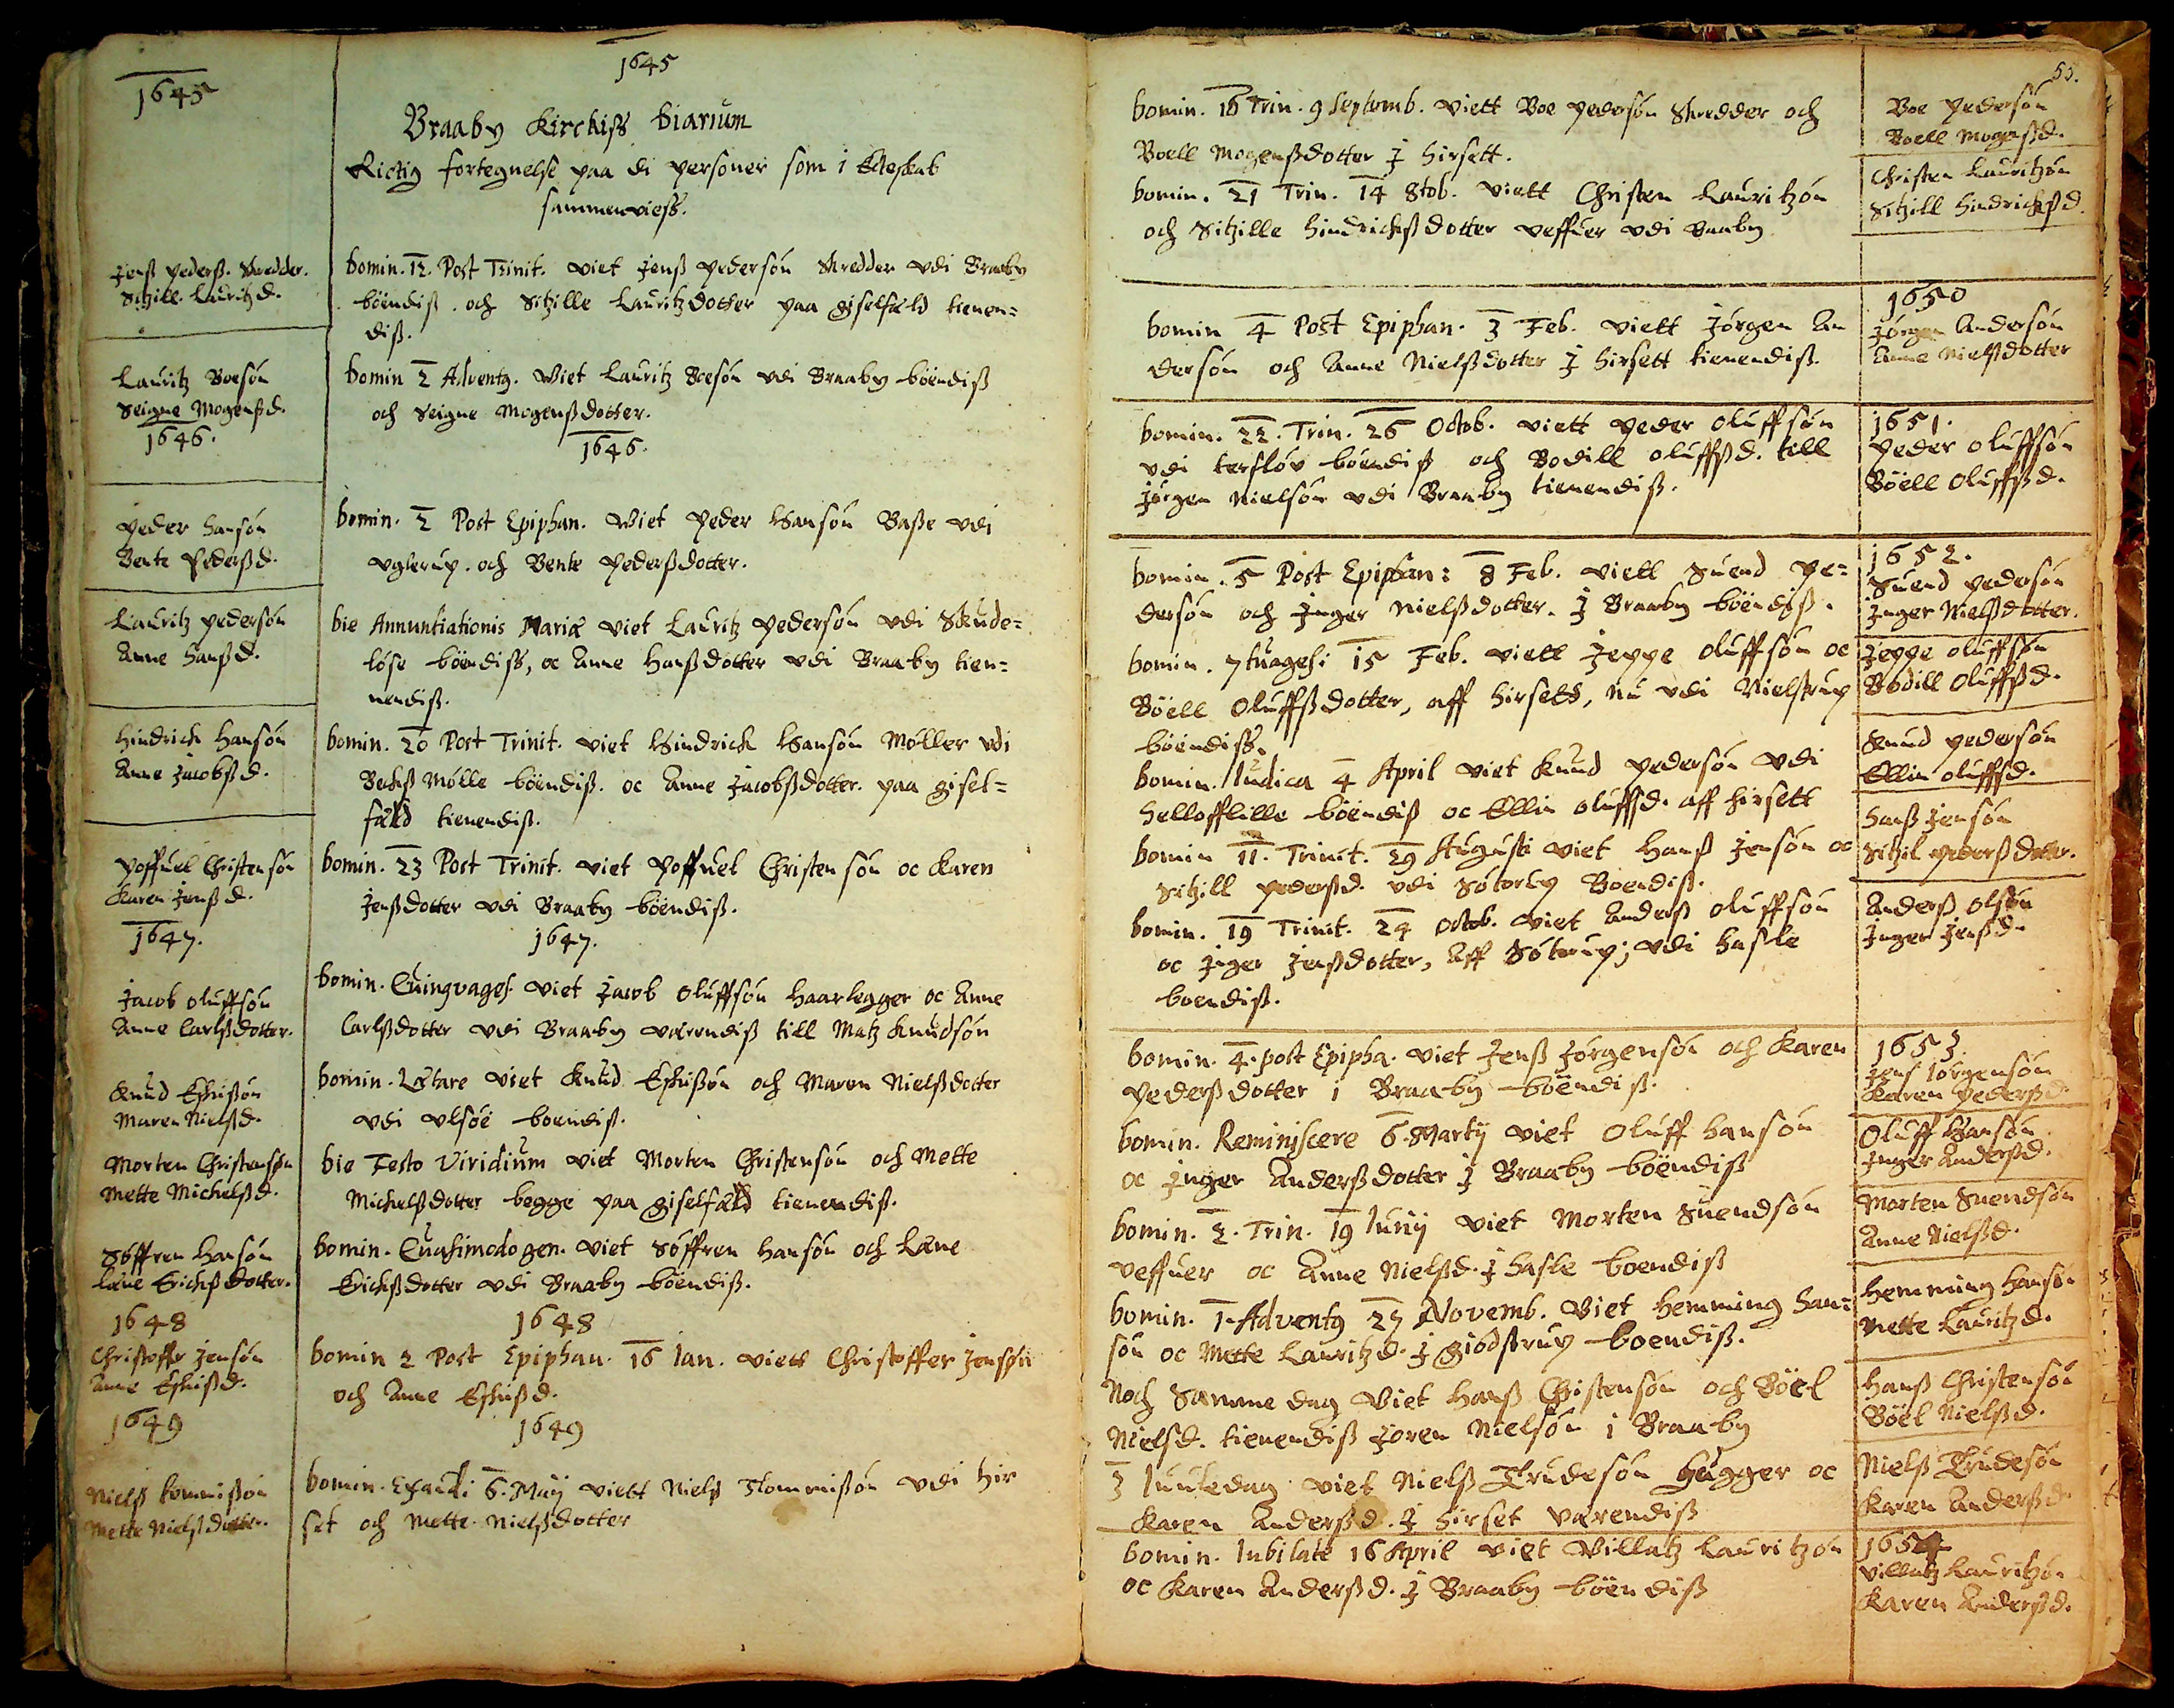1645 
1645 
Braaby Kirchiss Diarium 
Rictig fortegnelse paa di personer som i Ecteskab 
sammenviess.
Jens Peders. Skrædder.
Sitzill. Lauritzd.
Domin. 12. Post Trinit. Viet Jens Pedersøn Skredder Udi Braaby 
boindis och Sitzille Lauritzdotter paa Giselfeld tienen¬
dis. 
Lauritz Boesøn
Seigne Mogensd.
Domin 2 Adventy. Viet Lauritz Boesøn Udi Braaby boendis
och Seigne Mogensdotter. 
1646 
1646 
Peder Hansøn
Bente Pedersd.
Domin. 2 Post Epiphan. Viet Peder Hansøn Basse udi
Uglerup och Bente Pedersdotter.
Lauritz Pedersøn 
Anne Hansd.
Die Annuntiationis Mariæ Viet Lauritz Pedersøn Udi Skude¬
løse boendiss oc Anne Hansdatter Udi Braaby tien¬
nendis.
Hindrich Hansøn 
Anne Jacobsd.
Domin. 20. Post Trinit. Viet Hindrich Hansøn Møller Udi
Becks Mølle boendis. oc Anne Jacobsdotter paa Gisel¬
feld tienendis.
Poffuel Christensøn 
Karen Jensd. 
Domin. 23 Post Trinit. Viet Poffuel Christensøn oc Karen 
Jensdatter Udi Braaby boendis. 
1647.
1647.
Jacob Oluffsøn 
Anne Carlsdatter
Domin. Quinuages. Viet Jacob Oluffsøn Haarlegger## oc Anne
Carlsdotter Udi Braaby værendis till Matz Knudsøn.
Knud Eskisøn
Maren Nielsd.
Domin. Lætare Viet Knud Eskisøn och Maren Nielsdotter 
Udi Ulsøe boendis.
Morten Christensøn 
Mette Michelsd. 
Die Festo Viridium Viet Morten Christensøn och Mette 
Michelsdotter begge paa Giselfeld tienendis. 
Søffren Hansøn 
Læne Erichsdatter.
Domin. Quasimodo gen. Viet Søffren Hansøn och Læne 
Erichsdotter Udi Braaby boendis.
1648 
1648 
Christoffer Jensøn
Anne Eskisd. 
Domin 2 Post Epiphan. 16. Jan. Viett Christoffer Jensøn 
och  Anne Eskisd.
1649 
1649 
Niels Tommisøn 
Mette Nielsdotter.
Domin Exaudi 6. May Viett Niels Thommisøn Udi Hir
set och Mette Nielsdotter
Domin. 16. Trin. 9 Septemb. Viett Boe Pedersøn Skredder och
Boell Mogensdotter J Hirsett.
55.
Boe Pedersøn 
Boell Mogensd.
Domin. 21 Trin. 14 8tob. Viett Christen Lauritzøn 
och  Sitzille Hindrichsdotter Veffuer Udi Braaby.
Christen Lauritzøn 
Sitzille Hindrichsd.
Domin 4 Post Epiphan. 3 Feb. Viett Jørgen An
dersøn och Anne Nielsdotter J Hirsett tienendis.
1650 
Jørgen Andersøn 
Anne Nielsdotter
Domin. 22. Trin. 26 Octob. Viett Peder Oluffsøn 
Udi Tersløv boendis ## och Bodill Oluffsd till
Jørgen Nielsøn Udi Braaby tienendis.
1651 
Peder Oluffsøn 
Boell Oluffsd.
Domin. 5 Post Epiphan: 8 Feb. Viett Suend Pe¬
dersøn och Inger Nielsdotter J Braaby boendis. 
1652.
Suend Pedersøn
Inger Nielsdatter.
Domin. 7tuages: 15 Feb. Viett Jeppe Oluffsøn oc 
Boell Oluffsdotter, aff Hirsett, nu Udi Nielstrup
boendiss.
Jeppe Oluffsøn 
Bodill Oluffsd.
Domin. Judica 4 April Viet Knud Pedersøn Udi 
Hellofflille boendis oc Ellin Olufsd. aff. Hirsett.
Knud Pedersøn 
Ellin Oluffd.
Domin 11. Trinit. 29 Augusti Viet Hans Jensøn oc 
Sitzill Pedersd. Udi Søtorup Boendis.
Hans Jensøn 
Sitzil Pedersdatter. 
Domin. 19 Trinit. 24 Octb. Viet Anders Oluffsøn 
oc Inger Inger Jensd. Aff Søtorup, Udi Hasle
boendis.
Anders Olsøn 
Inger Jensd.
Domin. 4. Post Epiphan. Viet Jens Jørgensøn och Karen 
Pedersdotter i Braaby boendis.
1653
Jens Jørgensøn 
Karen Pedersd. 
Domin. Reminiscere 6. Marty Viet Oluff Hansøn 
oc Inger Andersdotter J Braaby boendis.
Oluff Hansøn 
Inger Andersd.
Domin. 2. Trin. 19 Juny Viet Morten Suendsøn 
Veffuer oc Anne Nielsd. J Hasle boendis. 
Morten Suendsøn 
Anne Nielsd.
Domin. 1. Adventy 27 Novemb. Viet Hemming Han¬
søn oc Mette Lauritzd. J Giodstrup boendis. 
Hemming Hansøn 
Mette Lauritzd.
Noch samme dag Viet Hans Christensøn och Boel 
Nielsd. tienendis Jøren Nielsøn i Braaby.
Hans Christensøn 
Boel Nielsd. 
3 Juuledag Viet Niels Trudesøn Hugger oc 
Karen Andersd. J Hirset værendis. 
Niels ##Trudesøn
Karen Andersd.
Domin. Jubilate 16 April Viet Villatz Lauritzøn 
oc Karen Andersd. J Braaby boendis.
1654
Villatz Lauritzøn 
Karen Andersd.
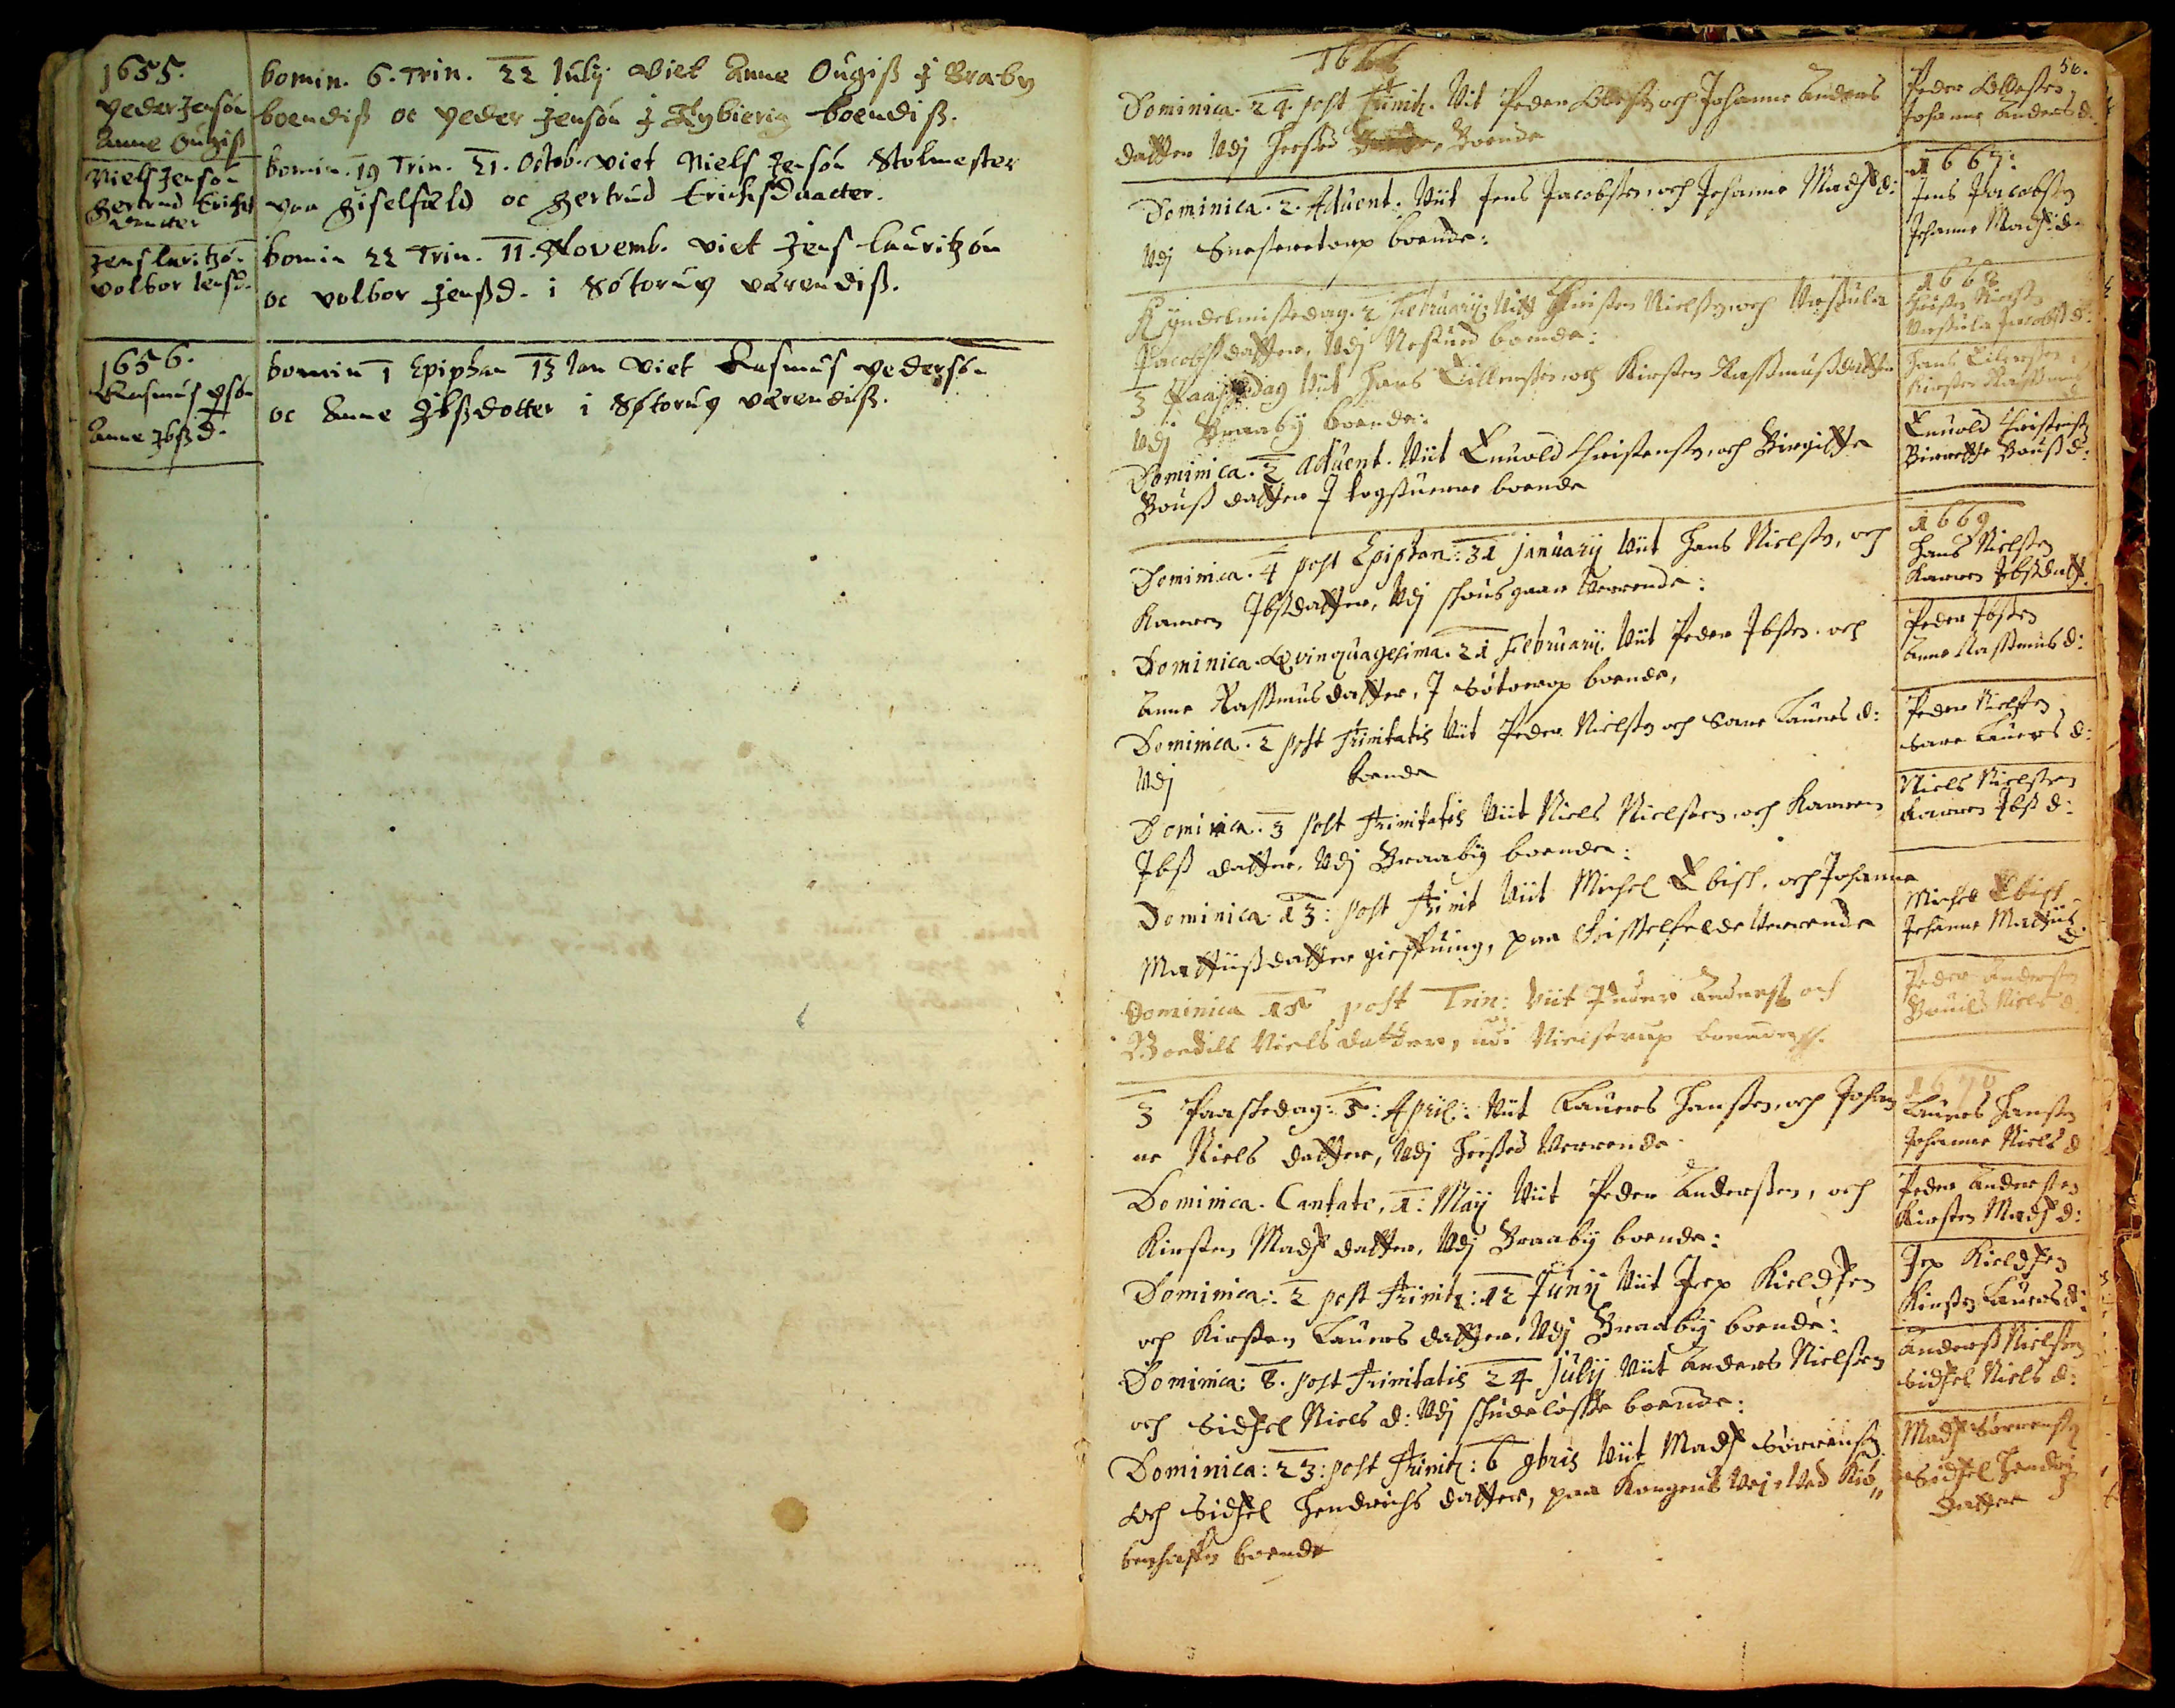1655 
Peder Jensøn 
Anne Ougis 
Domin. 6. Trin. 22 July Viet Anne Ougis J Braaby
boendis oc Peder Jensøn J Tybierig boendis. 
Niels Jensøn 
Gertrud Erichs
datter 
Domin. 19 Trin. 21. Octob. Viet Niels Jensøn Stalmester 
paa Giselfeld oc Gertrud Erichsdaatter. 
Jens Laritzøn
Valbor Jensd. 
Domin 22 Trin. 11.Novemb. Viet Jens Lauritzøn
oc Valbor Jensd. i Søtorup værendis.
1656
Rasmus Psøn
Anne Ibsd. 
Domin I Epiphan 13 Jan. Viet Rasmus Pedersøn 
oc Anne Jbsdatter i Søtorup værendis. 
1666
Dominica. 24. post Trinit. Vit Peder Ollesøn och Johanne Anders
datter. Udj Hersed ##, Boende.
56.
Peder Ollesøn 
Johanne Andersd:
Dominica. 2. Aduent: Viit Jens Jacobsen och Johanne Madsd:
Udi Sneseretorp boende:
1667
Jens Jacobsen
Johanne Madsd:
Kyndelmissedag. 2. February Vitt Christen Nielsøn och Ursula
Jacobsdatter. Udj Nestued boende:
1668 
Christen Niels
Ursula Jacobsd: 
3. Paaskedag Viit Hans Eillersøn og Kirsten Rassmusdatter. 
Udj Braaby boende: 
Hans Eilersøn 
Kirsten Rassmusd:
Dominica. 2 Aduent. Viit Enuold Christensen, och Birgitte
Boussdatter J Togsuerre boende.
Enuold Christensen
Birrette Bousd: 
Dominica. 4. post Epiphan: 31 January Viit Hans Nielsen och
Karren Jbsdatter, Udj Shousgaar værende:
1669
Hans Nielsen
Karren Ibsdatter 
Dominica. Quinquagesima. 21 Februarij. Viit Peder Jbssen och
Anna Rassmusdatter, J Søtoerup boende. 
Peder Ibsen
Anna Rassmusd:
Dominica. 2. post Trinitatis Viit Peder Nielsen och Sara Lauersd:
Udj
boende.
Peder Nielsen
Sara Laursd:
Dominica. 3 post Trinitatis Viit Niels Nielsen och Karren
Jbs datter. Udj Braaby boende.
Niels Nielsen
Karren Ibsd:
Dominica 13: post Trinit Viit Michel Ebiss, och Johanne 
Mathiisdatter giestning, paa Gisselfeld Verrende.
Michel Ebiss
Johanne Mathiisd:
Dominica 15. post Trin: Viit Peder Anders och
Boedill Nielsdatter, udi Nielstrup boendess. 
Peder Andersen
Bouild Nielsd:
3. Paaskedag 5: April: Viit Laures Hansen och Johan
ne Niels Datter, Udj Hirsed Verrende. 
1670
Laures Hanssøn 
Johanne Nielsd:
Dominica Cantate, 1: May Viit Peder Andersøn, och 
Kirsten Mads Datter, Udj Braaby boende:
Peder Andersen
Kirsten Madsd:
Dominica 2 post Trinit: 12 Juny Viit Jep Kieldsøn 
och Kirsten Lauers Datter. Udj Braaby boende: 
Jep Kieldsen
Kirsten Lauersd:
Dominica 8. post Trinitatis 24 July Viit Anders Nielsen
och Sidsel Nielsd: Udj Skudeløsse boende.
Anders Nielsen
Sidsel Nielsd: 
Dominica 23: post Trinit: 6. 9bris. Viit Mads Sørrensen
och Sidsel Hendrichs Datter, paa Kongens Vej Ved Kiø¬
benhafn boende. 
Madz Sørrensen
Sidsel Hendrichs
datter